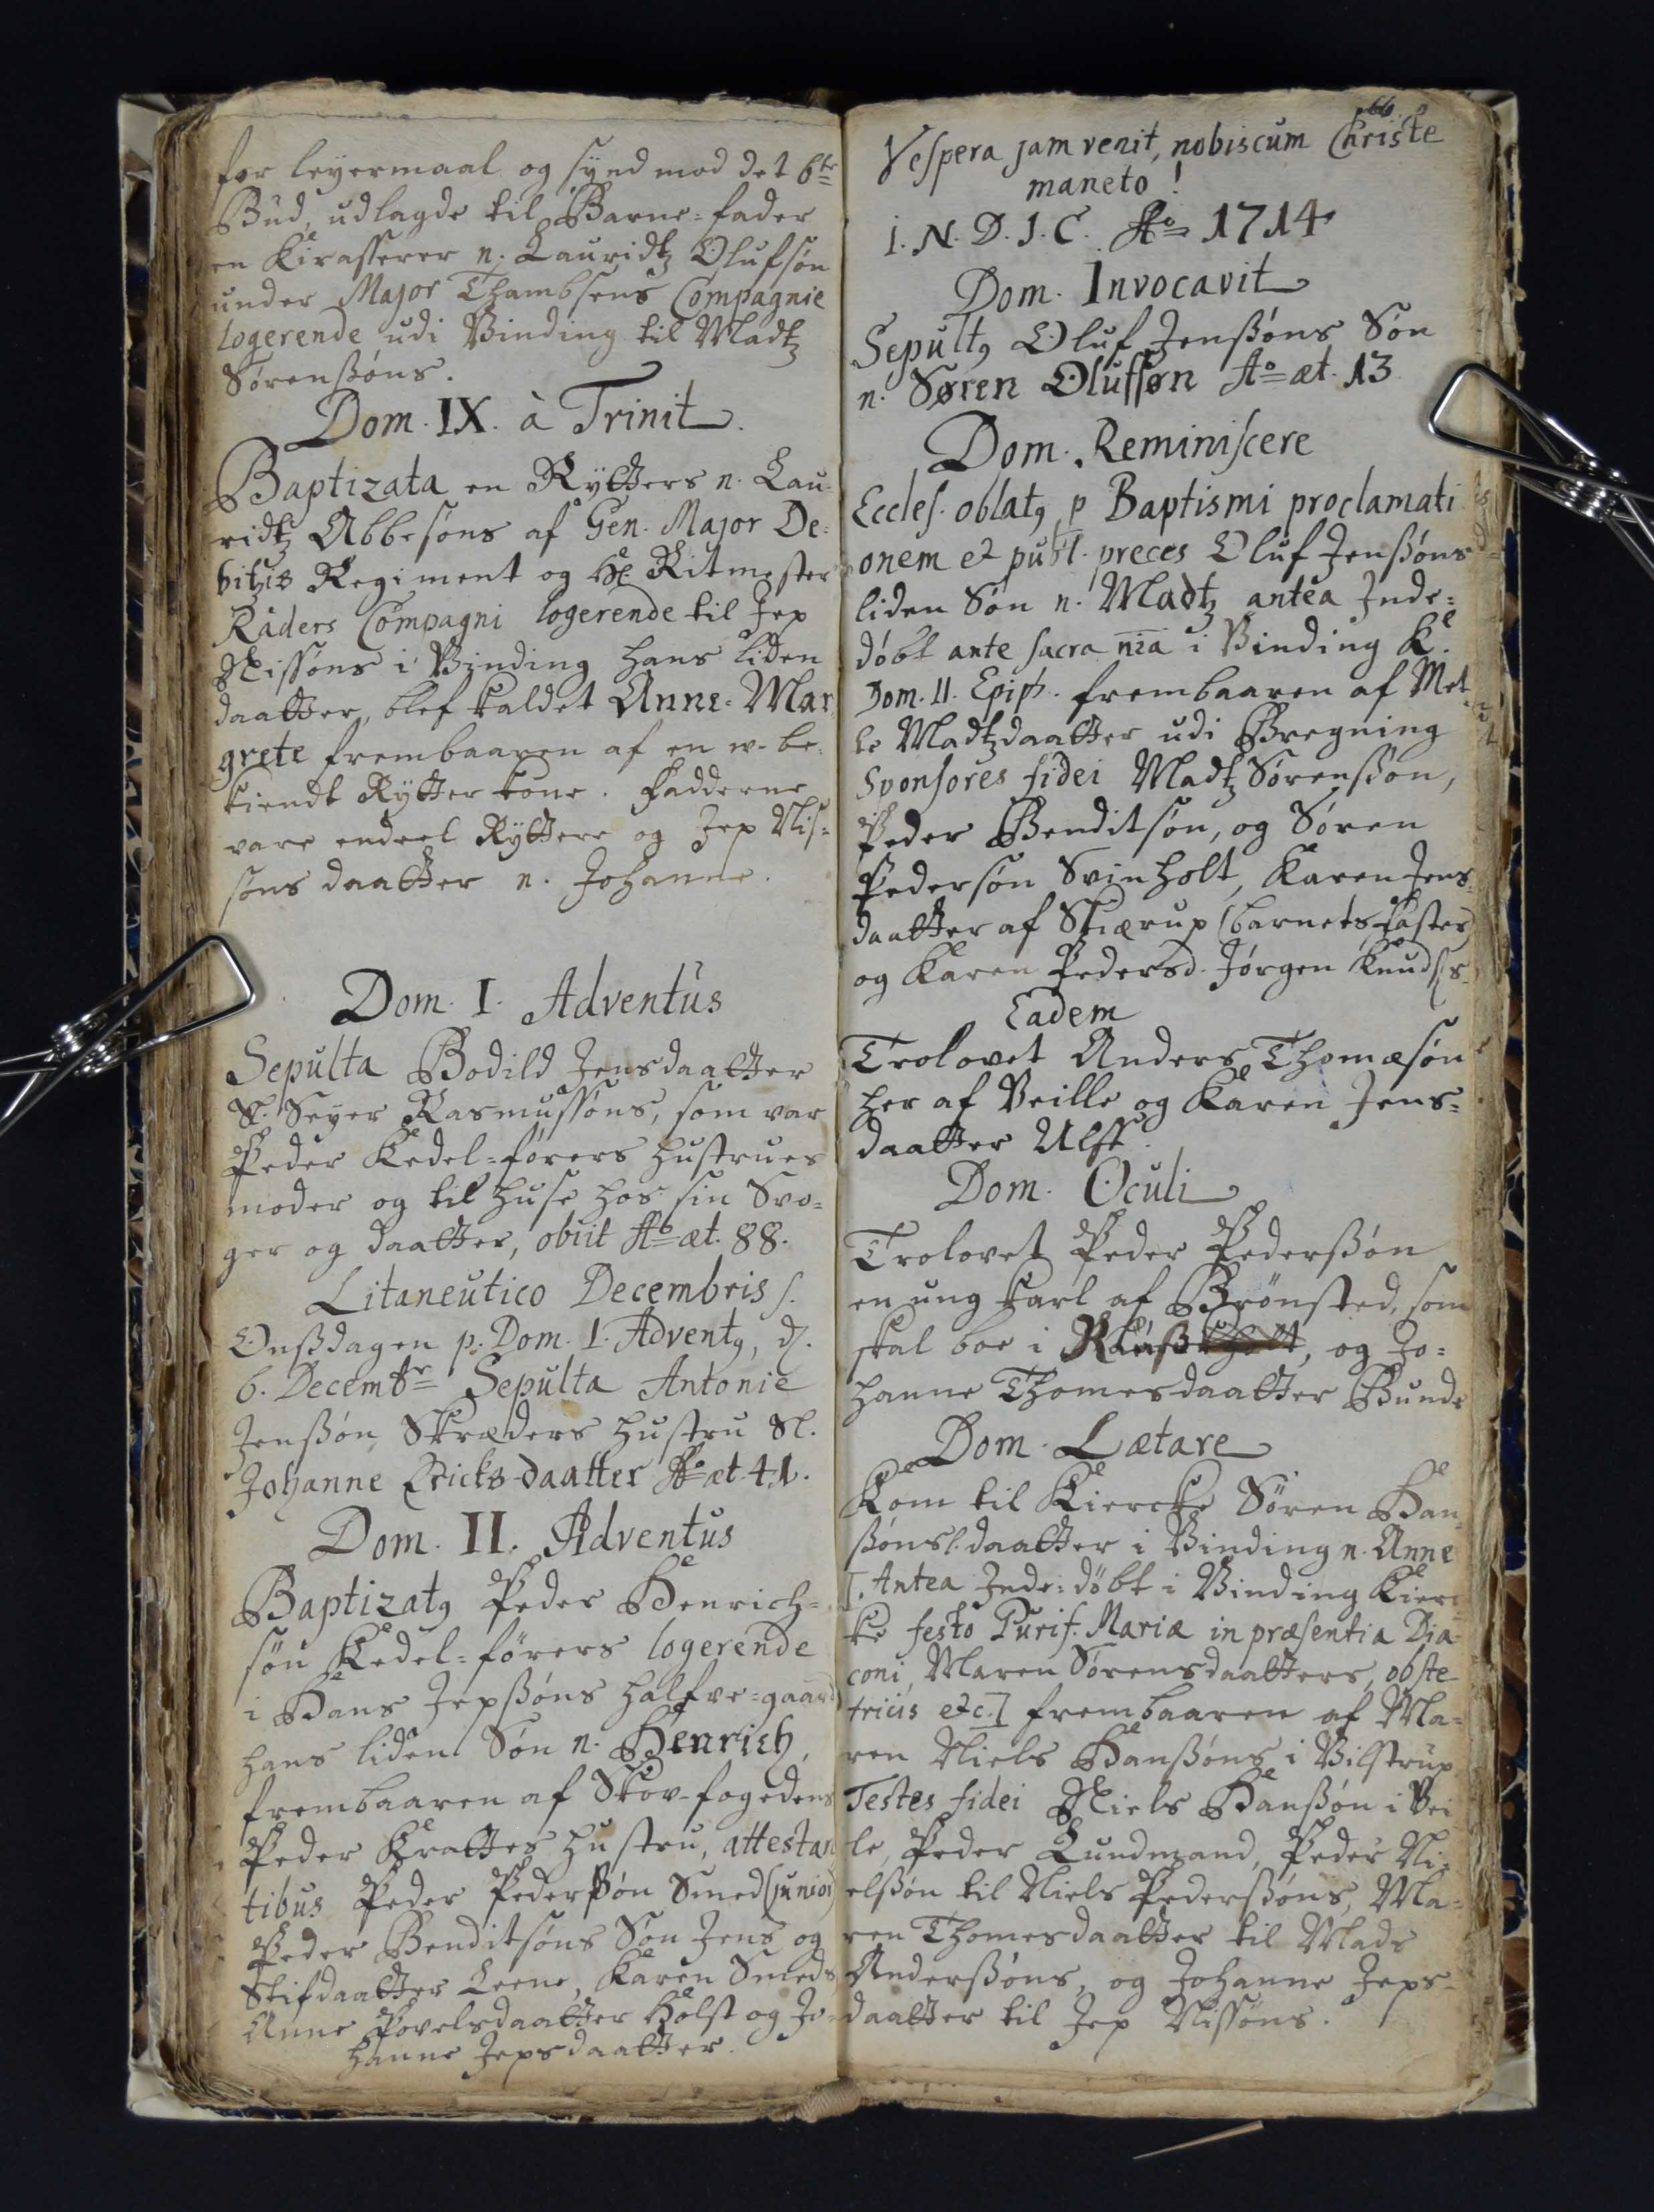for leyermaal og synd mod det 6te
Bud, udlagde til Barne=fader 
en Kirasserer n. Lauridtz Olufsøn
under Major Thambsens Compagnie
logerende udi Vinding til Madtz
Sørensøns
Dom. IX. a Trinit
Baptizata en Rytters n. Lau¬
ridtz Abbesøns af Gen. Major De¬
bitzis Regiment og Hl Ritmester
Raders Compagni logerende til Jep
Nissøns i Vinding hans liden
daatter, blef kaldet Anne=Mar¬
grete, frembaaren af en w-be¬
kiendt Rytter Kone. Fadderne 
vare endeel Ryttere og Jep Nis¬
søns daatter n. Johanne
Dom. I. Adventus
Sepulta Bodild Jensdaatter
Sl. Seyer Rasmussøns, som var
Peder Kedel=førers hustrues
moder og til huse hos sin Svo¬
ger og daatter, obiit Ao æt. 88.
Litaneutico Decembris s.
Onssdagen p. Dom. I. Adventq, d.
6. Decembr Sepulta Antonie
Jenssøn Skræders hustru Sl.
Johanne Ericks-daatter Ao æt. 41.
Dom. II. Adventus
Baptizatq Peder Henrich¬
søn Kedel=førers logerende
i Hans Jepssøns halfve=gaard
hans liden Søn n. Henrich
frembaaren af Skov-fogedens
Peder Krattes hustru, attestan
tibus Peder Pederssøn Smed (junior)
Peder Benditsøns Søn Jens og
Stifdaatter Leene, Karen Smeds
Anne Povelsdaatter Holst og Jo¬
hanne Jepsdaatter.
66
Vespera jamvenit, nobiscum Christe
maneto!
I. N. D. J. C. Ao 1714
Dom. Invocavit
Sepultq Oluf Jenssøns Søn
n. Søren Olufsøn Ao æt. 13
Dom. Reminiscere
Eccles. oblatq, p. Baptismi proclamati
omen et publ. preces Oluf Jenssøns
liden Søn n. Madtz antea Inde¬
døbt ante sacra nia i Vinding K. 
Dom. II. Epiph. frembaaren af Met¬
te Madtzdaatter udi Breining
Sponsores fidei Madtz Sørenssøn,
Peder Benditsøn, og Søren 
Pedersøn Svinholt, Karen Jens¬
daatter af Skiærup, (barnets faster)
og Karen Pedersd. Jørgen Knudss
Eadem
Trolovet Anders Thomæsøn
her af Veille og Karen Jens¬
daatter Ulff
Dom. Oculi
Trolovet Peder Pederssøn
en ung Karl af Brønsted, som
skal boe i R##ss ##, og Jo¬
hanne Thomasdaatter Bunde
Dom. Lætare
Kom til Kiercke Søren Han¬
ssøns l. daatter i Vinding n. Anne
[Antea Inde døbt i Vinding Kierc¬
ke festo Purif. Mariæ in præsentia Dia¬
coni, Maren Sørensdaatter, obste¬
tricis etc], frembaaren af Ma¬
ren Niels Hanssøns i Vilstrup
Testes fidei Niels Hanssøn i Vei 
le, Peder Lundmand, Peder Ni¬
elssøn til Niels Pederssøns, Ma¬
ren Thomasdaatter til Madz
Anderssøns, og Johanne Jeps¬
daatter til Jep Nissøns.
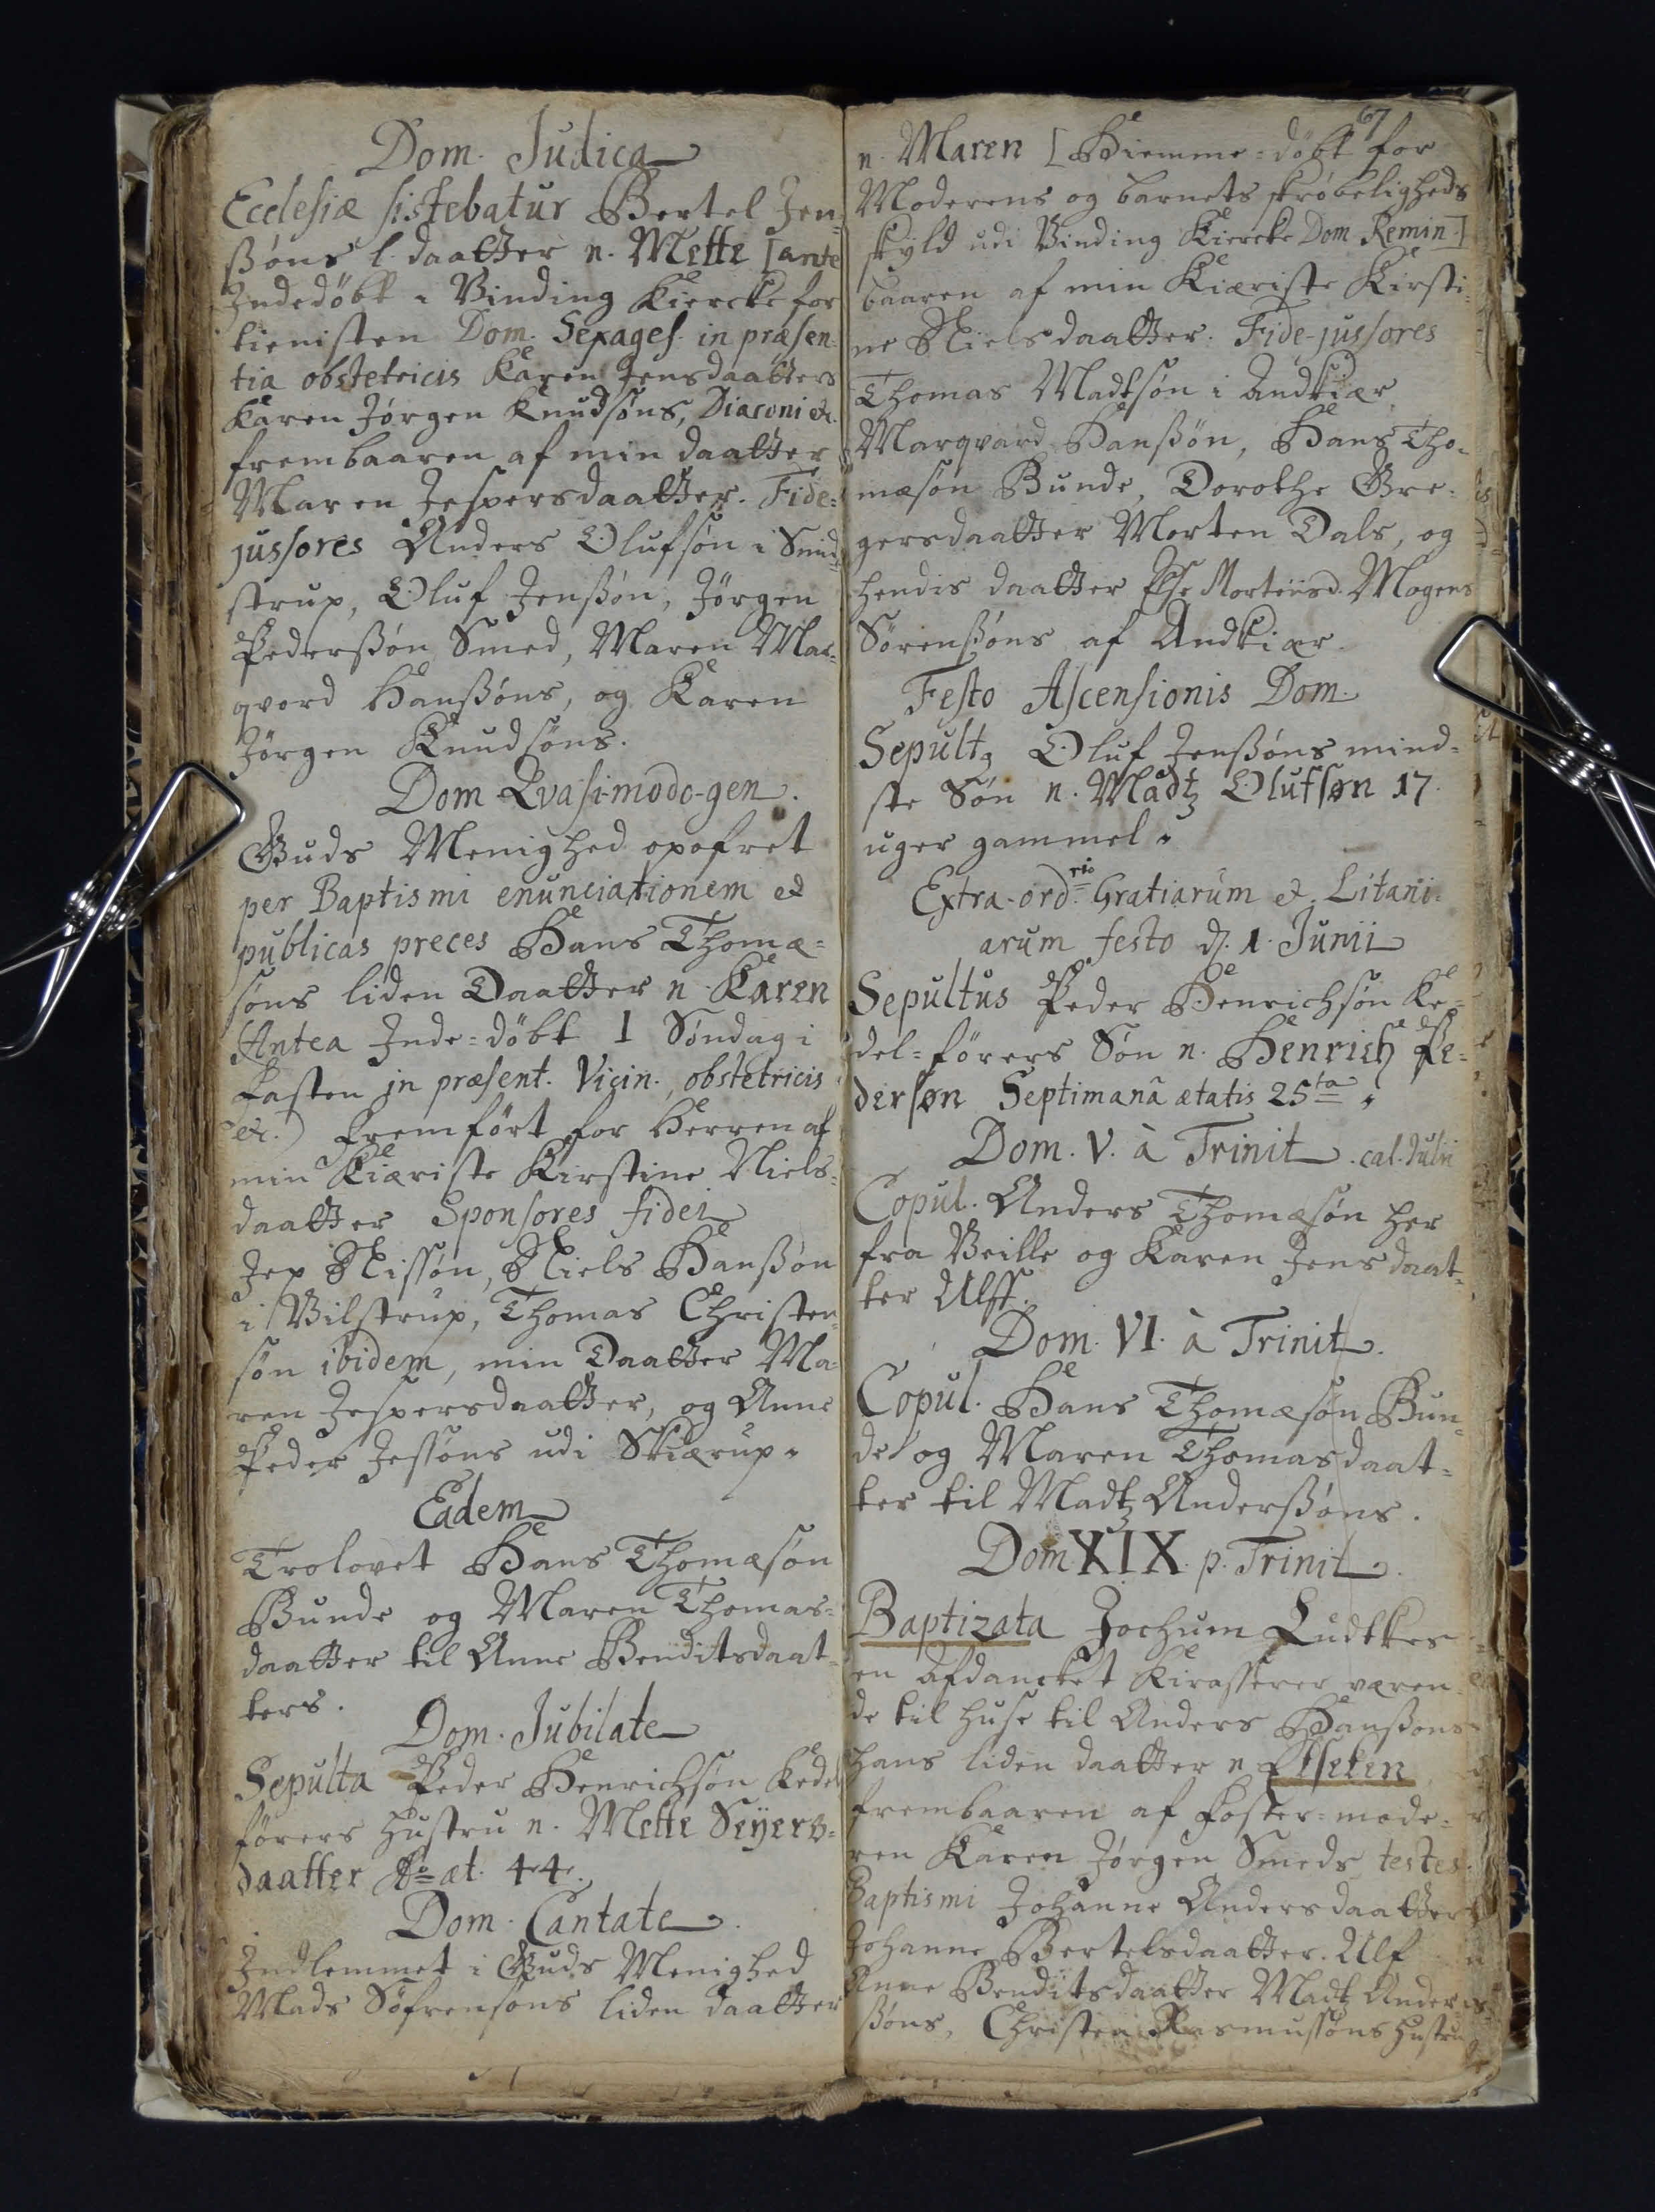Dom. Judica.
Ecclesiæ sistebatur Bertel Jen¬
ssøns l. daatter n. Mette [antea
Indedøbt i Vinding Kiercke for 
tienisten Dom. Sexages. in præsen¬
tia obstetricis Karen Jensdaatters
Karen Jørgen Knudsøns, Diaconi etc.
frembaaren af min daatter
Maren Jespersdaatter. Fide¬
jussores Anders Olufsøn i Smid¬
strup, Oluf Jenssøn, Jørgen
Pederssøn Smed, Maren Mar¬
qvard Hanssøns, og Karen
Jørgen Knudsøns.
Dom. Qvasi-modo-gen
Guds Menighed opofret
per Baptismi enunciationem et
publicas preces Hans Thomæ¬
søns liden Daatter n. Karen
(Antea Inde=døbt I Søndag i
Fasten in præsent. Vicin. obstetricis
etc.) fremført for Herren af
min Kiæriste Kirstine Niels¬
daatter Sponsores fidei
Jep Nissøn, Niels Hanssøn
i Vilstrup, Thomas Christen¬
søn ibidem, min Daatter Ma¬
ren Jespersdaatter, og Anne
Peder Jessøns udi Skiærup.
Eadem
Trolovet Hans Thomæsøn
Bunde og Maren Thomas¬
daatter til Anne Benditsdaat¬
ters.
Dom. Jubilate
Sepulta Peder Henrichsøn Kedel
førers hustru n. Mette Seyers¬
daatter Ao æt. 44
Dom. Cantate
Indlemmet i Guds Menighed 
Madtz Søfrensøns liden daatter 
67
n. Maren [Hiemme=døbt for
Moderens og barnets skrøbeligheds
skyld udi Vinding Kiercke Dom. Remin.]
baaren af min Kiæriste Kirsti¬
ne Nielsdaatter. Fide-jussores
Thomas Madtsøn i Andkiær
Marqvard Hanssøn, Hans Tho¬
mæsøn Bunde, Dorthe Gre¬
gersdaatter Morten Dals, og
hendis daatter Else Mortensd. Mogens
Sørenssøns af Andkiær.
Festo Ascensionis Dom.
Sepultq Oluf Jenssøns mind¬
ste Søn n. Madtz Olufsøn 17
uger gammel.
Extra-ord## Gratiarum et Litani¬
arum festo d. 1. Junii
Sepultus Peder Henrichsøn Ke¬
del=førers Søn n. Henrich Pe¬
dersøn Septimana ætatis 25ta
Dom. V. a Trinit. cal. Julee
Copul. Anders Thomæsøn her
fra Veille og Karen Jensdaat¬
ter Ulff
Dom. VI. a Trinit.
Copul. Hans Thomæsøn Bun¬
de og Maren Thomasdaat¬
ter til Madtz Anderssøns.
Dom. XIX. p. Trinit
Baptizata Jochum Ludtkes
en Afdancket Kirasserer væren¬
de til huse til Anders Hanssøns,
hans liden daatter n. Elseken
frembaaren af Foster=mode¬
ren Karen Jørgen Smeds testes
Baptismi Johanne Andersdaatter
Johanne Bertelsdaatter. Ulf
Anne Benditsdaatter Madtz Ander¬
ssøns, Christen Rasmussøns hustru